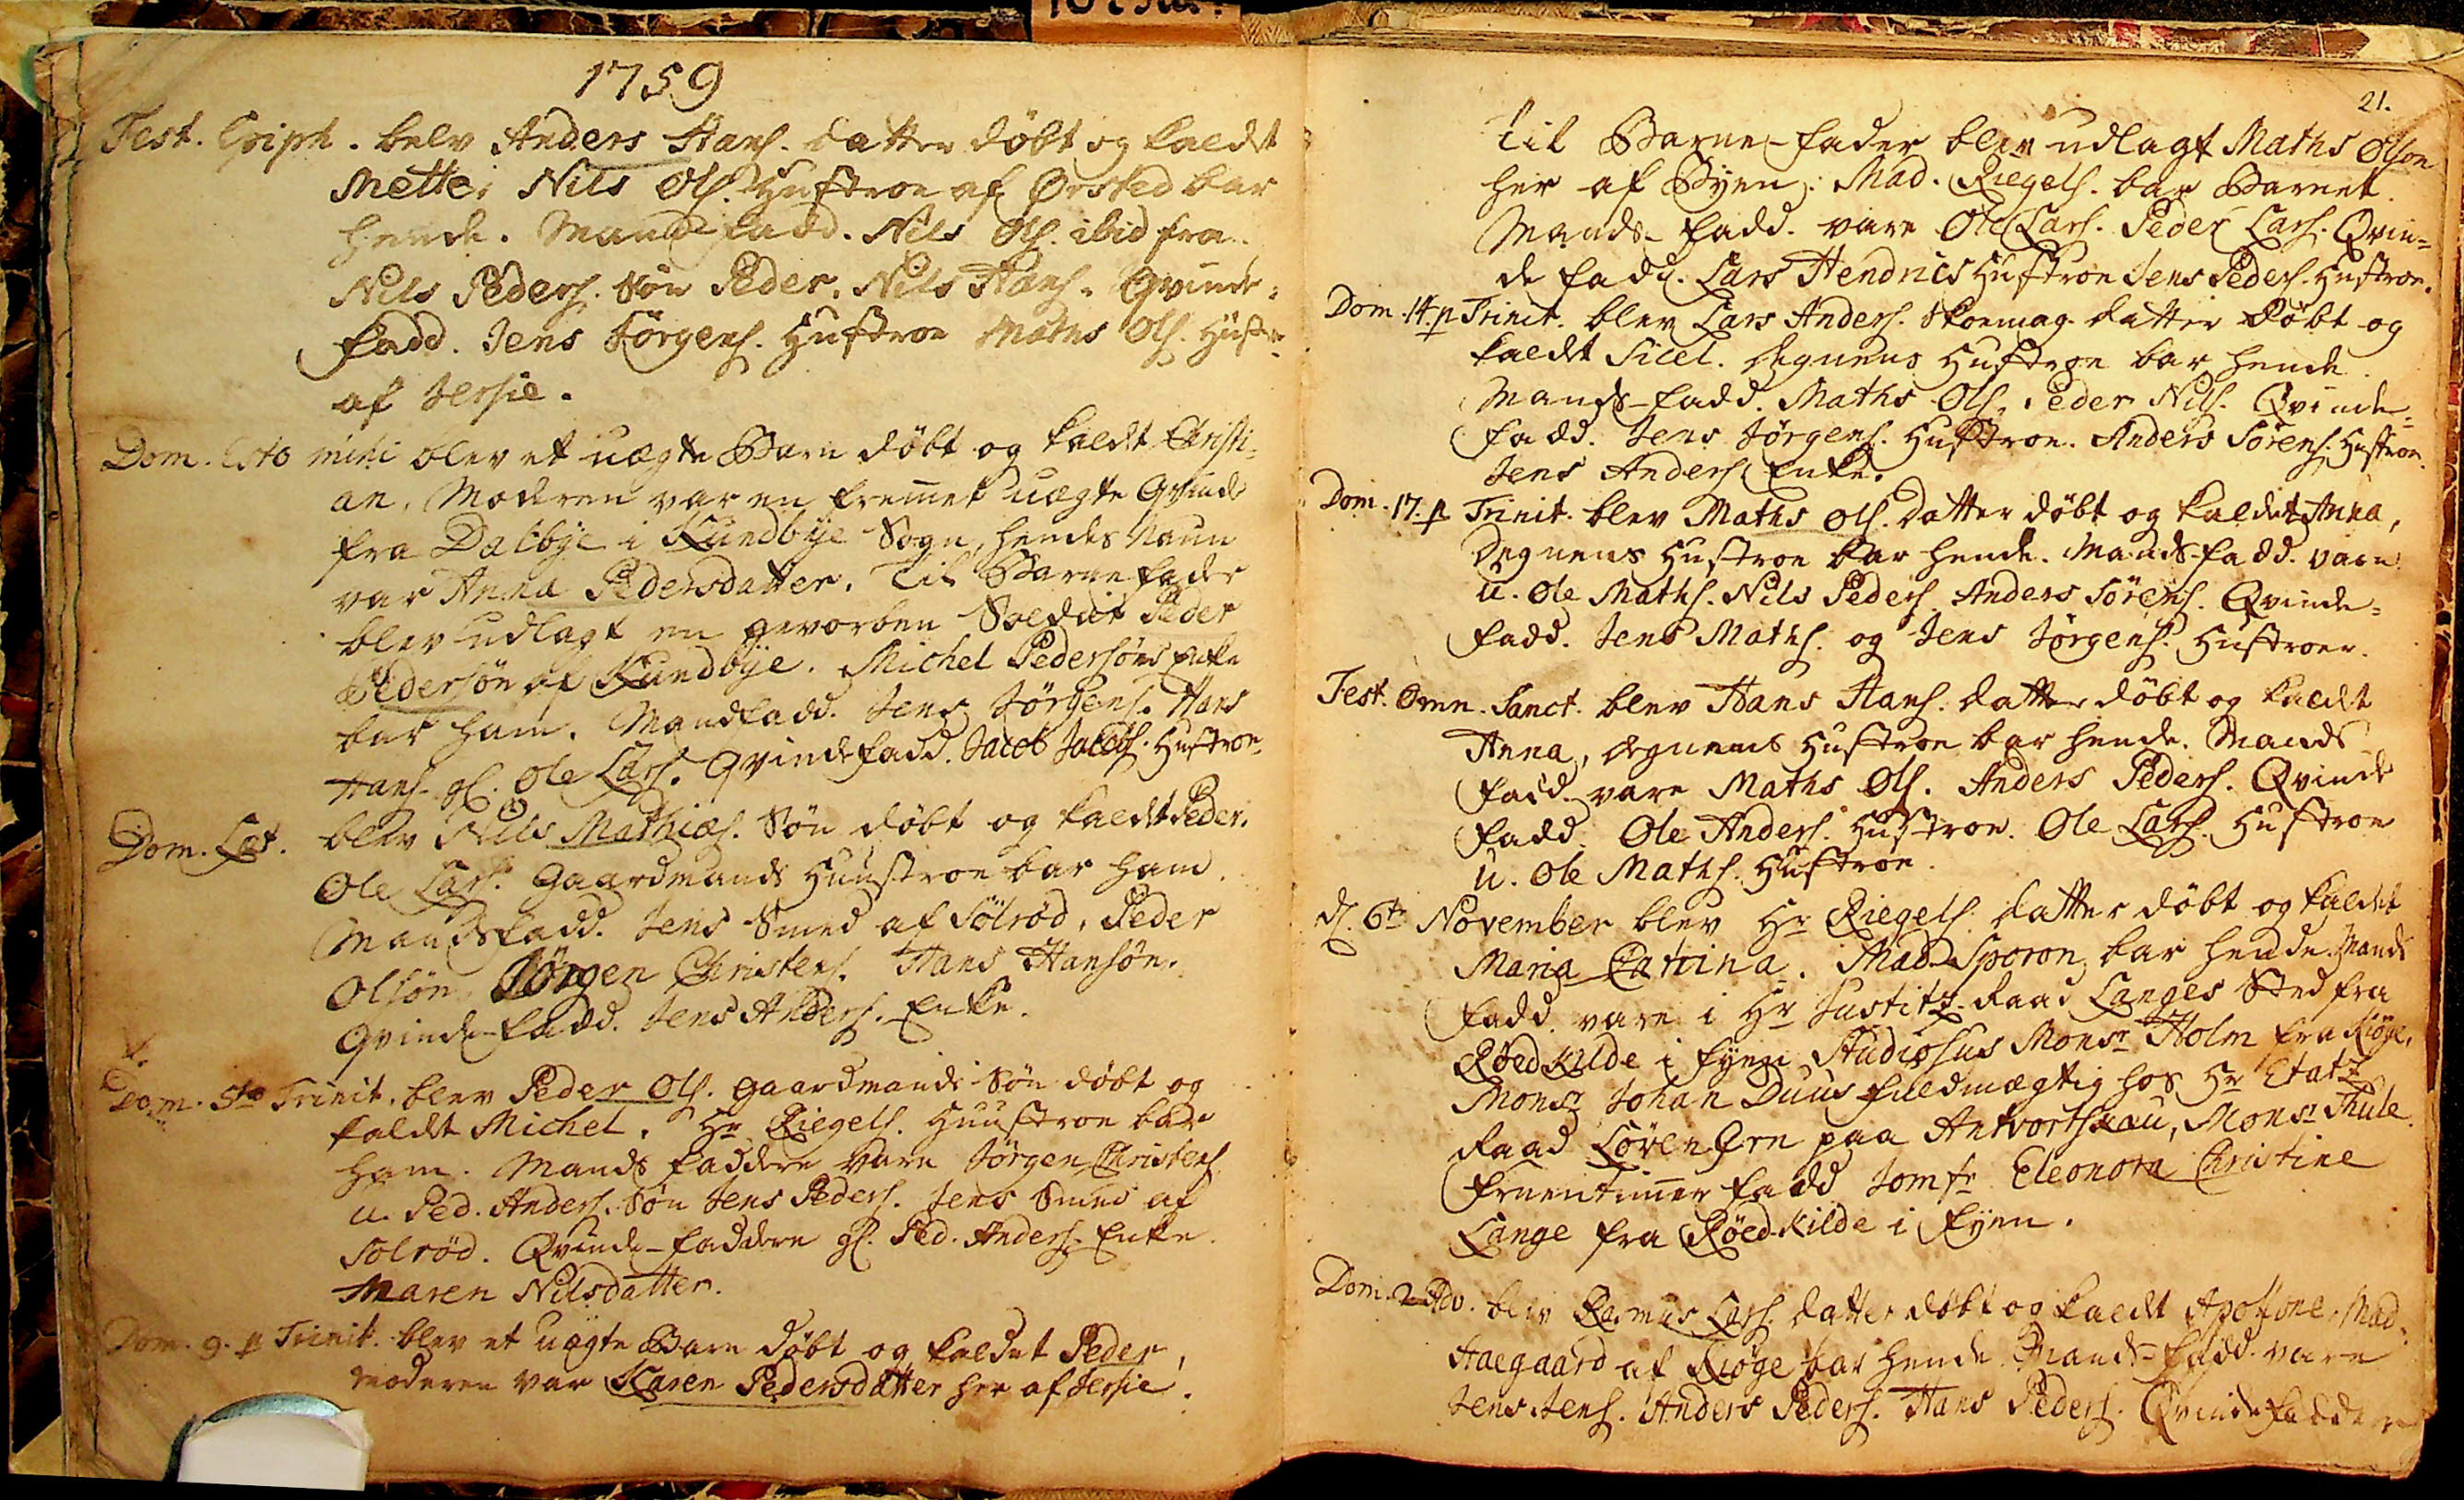1759
Fest. Epiph. belv Anders Hans. Datter døbt og kaldt
Mette, Nils Ols. Hustroe af Ørsted bar
hende. Mande fadd. Nils Ols. ibid fra
Nils Peders. Søn Peder. Nils Hans. Qvinde¬
fadd. Jens Jørgens. Hustroe  Maths Ols. Hustroe
af Jersie.
Dom. Esto mihi blev et uægte Barn døbt og kaldt Christi¬
an, Moderen var en fremmet uægte Qvinde
fra Dalbye i Kundbye Sogn, hendes Navn
var Anna Pedersdatter. Til Barnefader
blev udlagt en geworben Soldat Peder
Pedersøn af Kundbye. Michel Pedersøns Enke
bar ham. Mandfadd. Jens Jørgens. Hans
Hans. gl. Ole Lars. Qvindefadd. Jacob Jacobs. Hustroe.
Dom. Læt. blev Nils Mathiæs. Søn døbt og kaldt Peder.
Ole Lars. Gaardmands Hustroe bar ham.
Mandsfadd. Jens Smed af Solrød, Peder
Olsøn, Jørgen Christens. Hans Hansøn.
Qvinde-fadd. Jens Anders. Enke.
Dom. 5te Trinit. blev Peder Ols. Gaardmands Søn døbt og
kaldt Michel, Hr Riegels. Hustroe bar
ham. Mands faddere vare Jørgen Christens.
u. Ped. Anders. Søn Jens Peders. Jens Smed af
Solrød. Qvinde-faddere gl. Ped. Anders. Enke.
Maren Nilsdatter.
Dom. 9. p Trinit. blev et uægte Barn døbt og kaldet Peder,
Moderen var Karen Pedersdatter her af Jersie.
21.
til Barne-fader blev udlagt Maths Olson
her af Byen: Mad. Riegels. bar Barnet
Mands-fadd. vare Ole Lars. Peder Lars. Qvin¬
de fadd. Lars Hendrics Hustroe Jens Peders. Hustroe
Dom. 14. p Trinit. blev Lars Anders. Skoemag. datter Døbt og
kaldt Sicel. Degnens Hustroe bar hende.
Mands-fadd. Maths Ols. Peder Nils. Qvinde¬
fadd. Jens Jørgens. Hustroe. Anders Sørens. Hustroe
Jens Anders. Enke.
Dom. 17. p Trinit. blev Maths Ols. Datter døbt og kaldet Anna,
Degnens Hustroe bar hende. Mands-fadd. vare
U. Ole Maths. Nils Peders. Anders Sørens. Qvinde¬
fadd. Jens Maths. og Jens Jørgens. Hustroer.
Fest. Omn. Sanct. blev Hans Hans. Datter døbt og kaldt
Anna, Degnens Hustroe bar hende. Mands¬
fadd. vare Maths Ols. Anders Peders. Qvinde
fadd. Ole Anders. Hustroe. Ole Lars. Hustroe
U. Ole Maths. Hustroe.
d. 6te November blev Hr Riegels datter døbt og kaldet
Maria Catrina. Mad. Sporon bar hende. Mands
fadd vare i Hr Justitz-Raad Langes Sted fra
Røedkilde i Fyen Studiosus Monsr Holm fra Kiøge,
Monsr Johan Duus fuldmægtig hos Hr Etatz¬
Raad Løvenørn paa Antvortskou, Monsr Thule.
Fruentimmer fadd Jomfr Eleonora Christine
Lange fra Røedkilde i Fyen.
Dom.2.Adv. blev Rasmus Lars. Datter døbt og kaldt Apollone, Mad.
Aaegaard af Kiøge bar hende. Mands-fadd. vare
Jens Jens. Anders Peders. Hans Peders. Qvindefaddere vare